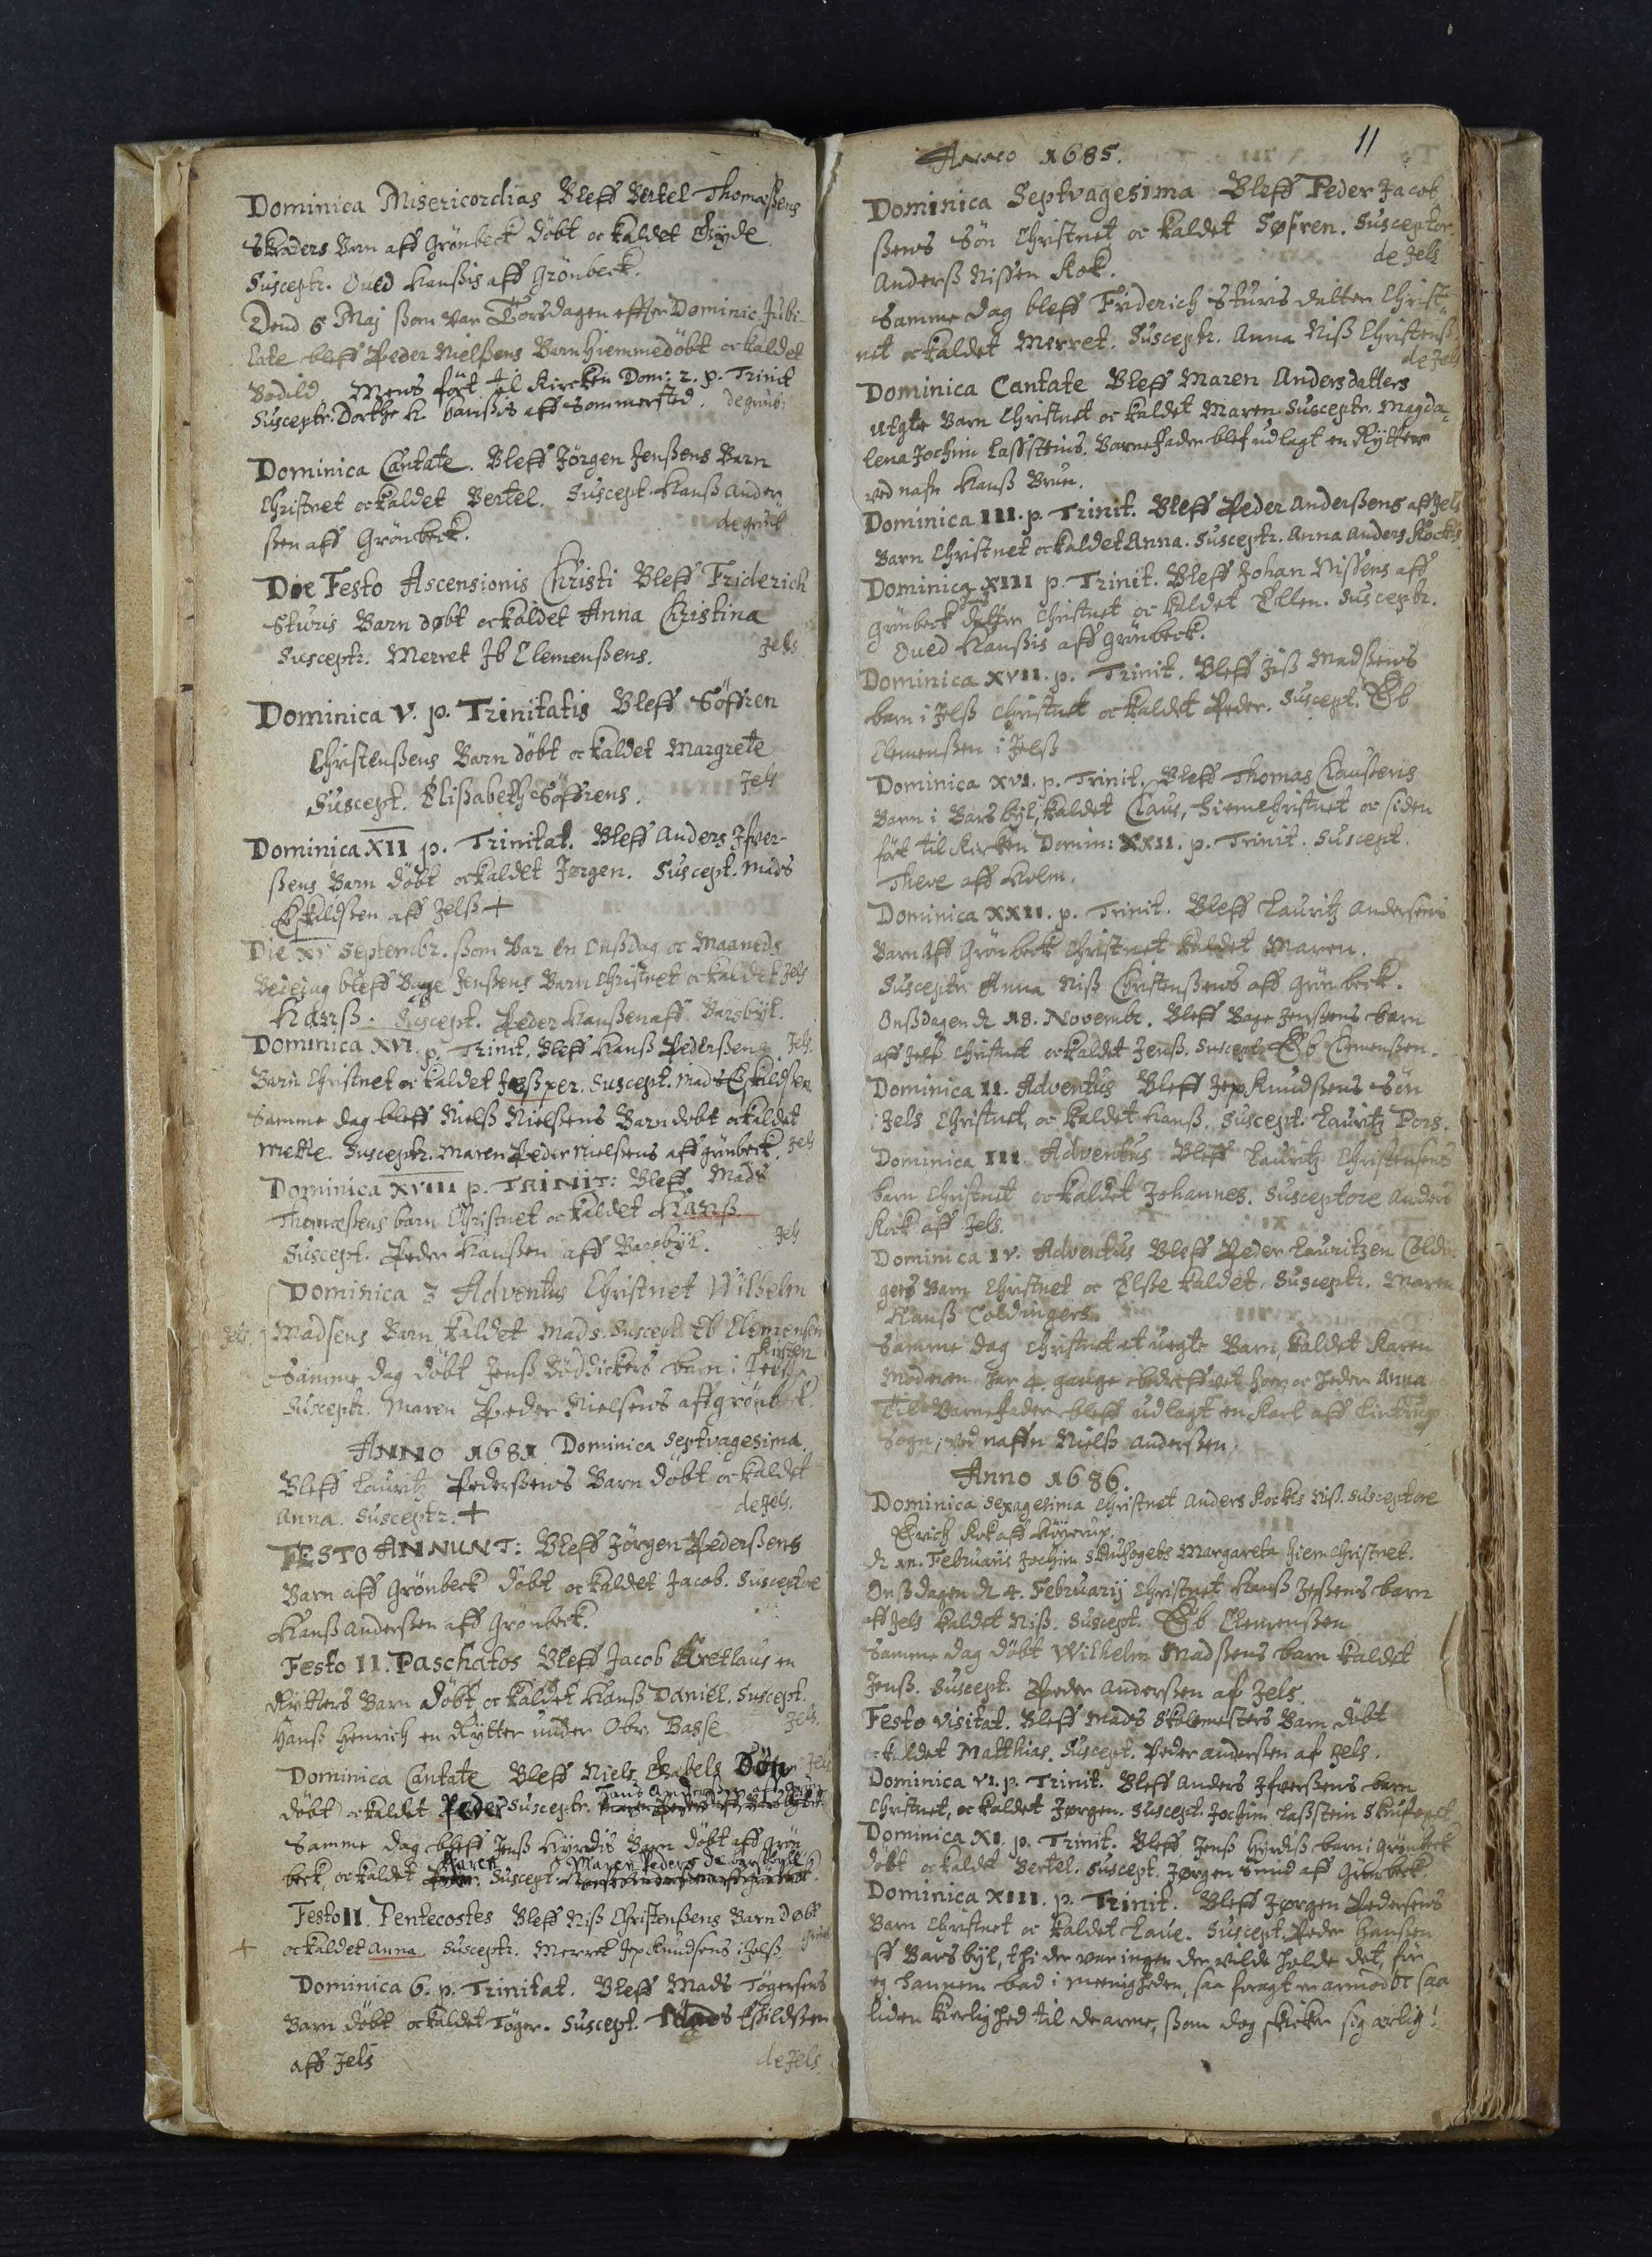Dominica Misericordias Bleff Bertel Thomasens
Skraders Barn aff Grønbeck døbt oc kaldet Gyde.
Susceptr. Oued Hansis aff Grønbeck.
Dend 6 Maj som var Torsdagen effter Dominic. Jubi¬
late bleff Peder Nielsens Barn Hiemmedøbt oc kaldet
Bodild. mens ført til Kircken Dom: 2. p. Trinit
Susceptr. Dorthe H Hansis aff Sommersted. de Grønb:
Dominica Cantate. Bleff Jørgen Jensens Barn
christnet oc kaldet Bertel. Suscept Hans Ander¬
sen aff Grønbeck.
De Grønb.
Die Festo Ascensionis Christi Bleff Friderich
Sturis Barn døbt oc kaldet Anna Christina
Susceptr. Merret Ib Clemensens.
Jels.
Dominica V. p. Trinitatis Bleff Søfren
Christensens Barn døbt oc kaldet Margrete
Suscept. Elisabeth Søffrens.
Jels.
Dominica XII p. Trinitat. Bleff Anders Ifer¬
sens Barn døbt oc kaldet Jørgen. Suscept. Mads
Eskildsen aff Jels.
Die XV Septembr. Som var en Onsdag oc Maaneds
Bededag bleff Boye Jensens Barn christnet oc kaldet Jels
Hanss. Suscept. Peder Hanssen aff Barsbyl.
Dominica XVI p. Trinit. Bleff Hans Pedersens Jels.
Barn christnet oc kaldet Jessper. Suscept. Mads Eskildsen.
Samme dag bleff Niels Nielsens Barn dobt oc kaldet
Mette. Susceptr. Maren Peder Nielsens aff Grønbeck. Jels
Dominica XVIII p. Trinit: Bleff Mads
Thomæssens barn christnet oc kaldet Hanss.
Suscept. Peder Hansen aff Barsbyl. Jels
Jels
Dominica 3 Adventus Christnet Wilhelm
Madsens Barn kaldet Mads. Suscept Ib Clemensen.
Kirsten
Samme dag døbt Jens Bøddickers barn i Jels
Susceptr. Maren Peder Nielsens aff Grønbeck.
ANNO 1681 Dominica Septvagesima.
Bleff Lauritz Pedersens Barn døbt oc kaldet
Anna Susceptr.
de Jels.
FESTO ANNUNT: Bleff Jørgen Pedersens
Barn aff Grønbeck døbt oc kaldet Jacob. Suscept.
Hans Andersen aff Grønbeck.
Festo II Paschatos Bleff Jacob Kretlaus en
Rütters Barn døbt oc kaldet Hanss Daniel. Suscept.
Hans Henrich en Rÿtter under Obr. Basse Jels.
Dominica Cantate Bleff Niels Gabels Søn## Jels
døbt oc kaldet Peder. Susceptr.
Hans Andersen af Grøn
Samme dag bleff Jens Hyrdis Barn døbt aff Grøn
beck, oc kaldet
Karen
Suscept.
Maren Peders de Barsbyll
Festo II. Pentecostes Bleff Niss Christensens Barn døbt
oc kaldet Anna. Susceptr. Merret Jep Knudsens i Jelss Grøn
Dominica 6. p. Trinitat. Bleff Mads Tøgersens
Barn døbt oc kaldet Tøger. Suscept. Mads Eskildsen
aff Jels.
de Jels
11
ANNO 1685.
Dominica Septvagesima Bleff Peder Jacob
sens Søn christnet oc kaldet Søfren. Susceptor.
Anders Nissen Kok.
de Jels
Samme dag bleff Friderich Sturis datter christ¬
net oc kaldet Merret. Susceptr. Anna Niss Christenss
de Jels
Dominica Cantate Bleff Maren Andersdatters
uegte Barn christnet oc kaldet Maren. Susceptr. Magda¬
lena Jochim Lasstrins. Barnefader blef udlagt en Rÿtter
ved nafn Hanss Brun.
Dominica III p. Trinit. Bleff Peder Andersens aff Jels
Barn christnet oc kaldet Anna. Susceptr. Anna Anders Kockis.
Dominica XIII p. Trinit. Bleff Johan Nissens aff
Grønbeck datter christnet oc kaldet Ellen. Susceptr.
Oued Hansis aff Grønbeck.
Dominica XVII p. Trinit. Bleff Jess Madssens
barn i Jels christnet oc kaldet Peder. Suscept. Ib
Clemensen i Jels
Dominica XVI. p. Trinit. Bleff Thomas Clausens
Barn I Barsbyl, kaldet Claus, hiemechristnet oc siden
ført til Kirken Domin: XXII. p. Trinit. Suscept.
Theve aff Holm.
Dominica XXII. p. Trinit. Bleff Lauritz Andersens
Barn aff Grønbeck christnet kaldet Maren.
Susceptr. Anna Niss Christenssens aff Grønbeck.
Onsdagen d 18. Novembr. Bleff Bage Jensens Barn
aff Jelss christnet oc kaldet Jenss. Suscept Ib Clemensen.
Dominica II. Adventus Bleff Jep Knudsens Søn
i Jels christnet oc kaldet Hanss. Suscept. Lauritz Pors.
Dominica III Adventus Bleff Lauritz Christensens
barn christnet oc kaldet Johannes. Susceptore Anders
Kork aff Jels.
Dominica IV. Adventus Bleff Peder Lauritzen Coldin¬
gers Barn christnet oc Elsse kaldet. Susceptr. Maren
Hanss Coldingers.
Samme Dag christnet et uegte Barn, kaldet Karen
Moderen har 4 gange bedreffvet hor oc Heder Anna
Til Barnefader bleff udlagt en karl aff Lintrup
Sogn; ved naffn Nielss Andersen.
Anno 1686.
Dominica sexagesima christnet Anders Kocks Niss. Susceptore
Erich kok aff Høyrup.
d xn.## Februaris Jochim Skouffogets Margareta hiemchristnet.
Onssdagen d 4. Februarij christnet Hans Jessens barn
aff Jels kaldet Niss. Suscept. Ib Clemensen
Samme Dag døbt Wilhelm Madsens barn kaldet
Jenss. Suscept. Peder Anderssen af Jels.
Festo Visitat. Bleff Mads Skolemesters Barn døbt
oc kaldet Matthias. Suscept. Peder Andersen af Jels.
Dominica VI. P. Trinit. Bleff Anders Iferssens barn
christnet, oc kaldet Jørgen. Suscept. Jochim Lasstein Skufoget
Dominica XI. p. Trinit. Bleff Jens Hyrdiss barn i Grønbeck
døbt oc kaldet Bertel. Suscept. Jørgen Smid aff Grønbeck.
Dominica XIII. p. Trinit. Bleff Jørgen Pedersens
Barn christnet oc kaldet Laue. Suscept. Peder Hansen
aff Barsbyl, thi der var ingen der vilde holde det, før
eg hannem bad i meenigheden, saa foragt er armod oc saa
liden kierlighed til de arme, som dog skicker sig arlig!